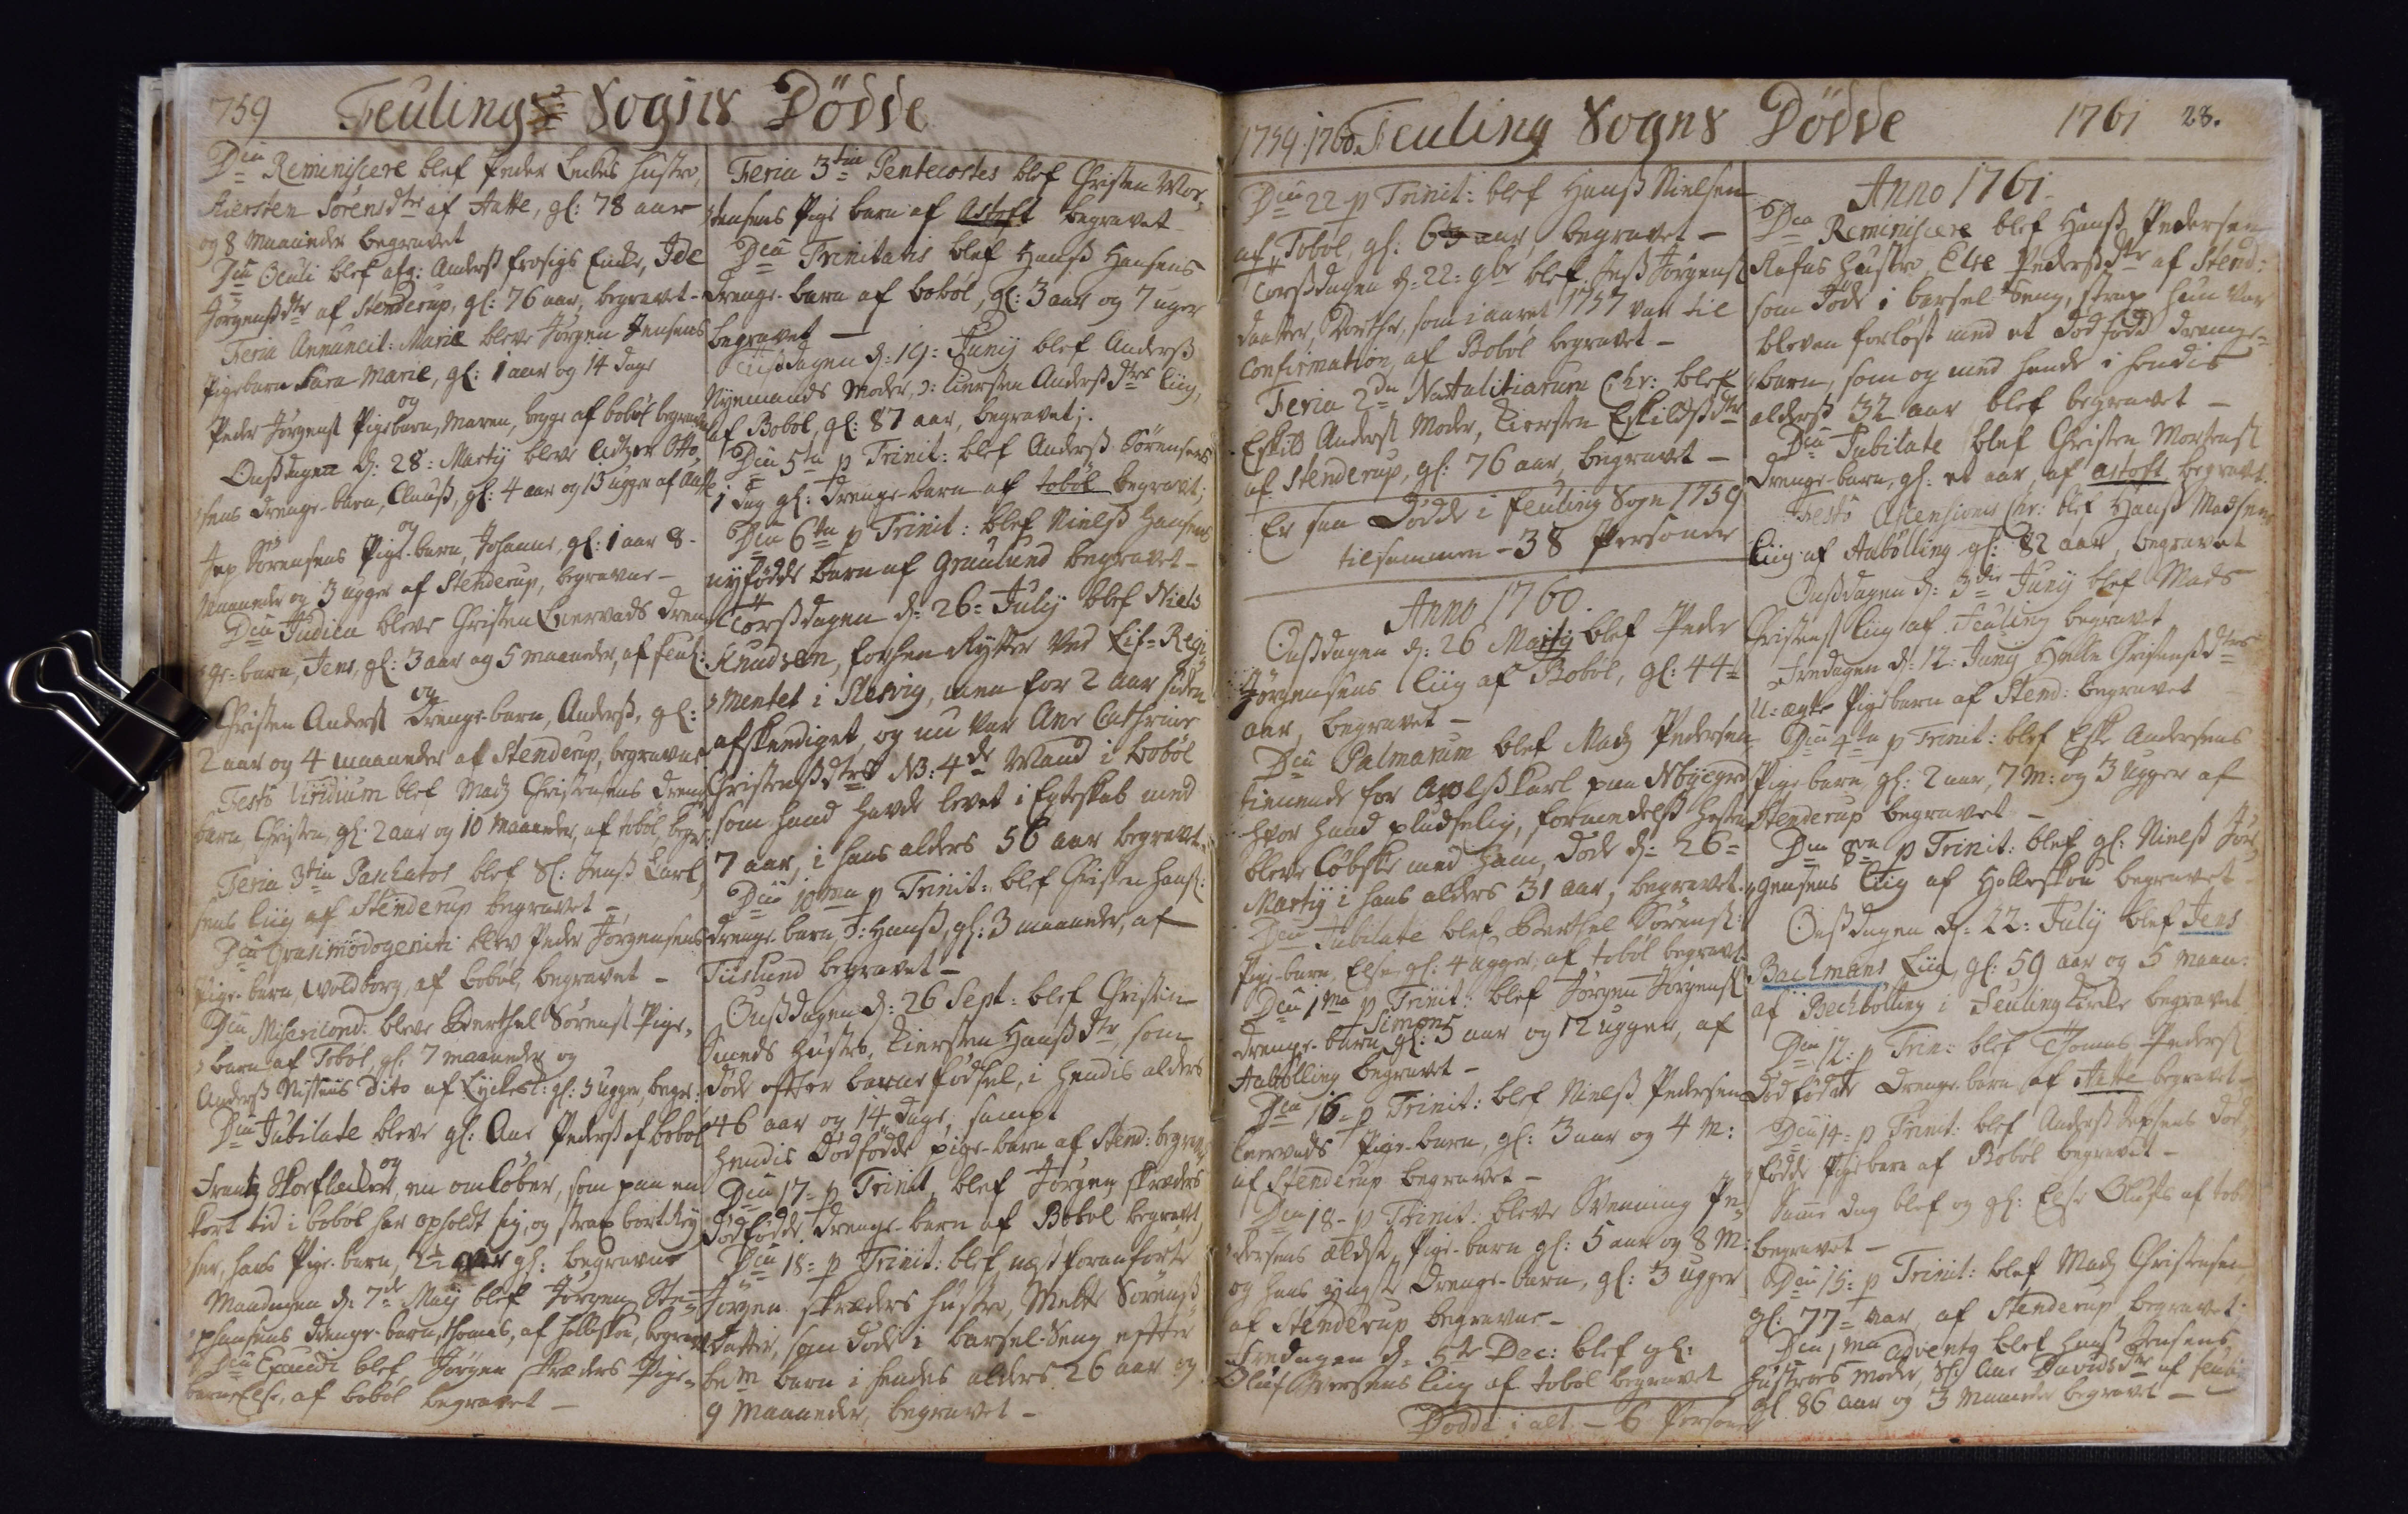1759 
Feulings  Sogns Dødde
Dca Reminiscere blef Peder Leckes Hustro
Kiersten Sørensdtr af Aatte, gl: 78 aar
og 8 Maaneder begravet
Dca Oculi blef afg: Anderss Frosigs Encke, Ide
Jørgensdtr af Stenderup, gl: 76 aar. begravet -
Feria Annuncit: Mariæ bleve Jørgen Jensens
Pigebarn Sara Marie, gl: 1 aar og 14 dage
og
Peder Jørgens Pigebarn, Maren, begge af bobøl begravne
Onssdagen d: 28: Martij bleve Adtzer Otto¬
sens Drenge-barn Clauss, gl: 4 Aar og 13 ugger af Aatte
og
Jep Sørensens Pige-barn, Johanne, gl: 1 aar 8 -
Maaneder og 3 ugger af Stenderup begravne -
Dca Judica bleve Christen Leervads Dren¬
ge=barn, Jens gl: 3 aar og 5 Maaneder, af Feul:
og
Christen Anders Drenge-barn Anderss, gl:
2 Aar og 4 maaneder af Stenderup begravne
Festo Viridium blef Madz Christensens Drenge
barn, Christen, gl: 2 Aar og 10 Maaneder, af tobøl begr:
Feria 3tia Paschatos blef Sl: Jens Carl
sens Liig af Stenderup begravet
Dca Qvasimodogeniti blev Peder Jørgensens
Pige-barn, Voldborg, af bobøl begravet -
Dca Misericord: bleve Berthel Sørens Pige¬
= barn af Tobøl, gl: 7 maaneder og
Anders Nissens Dito af Lyckesk: gl: 5 ugger, begr:
Dca Jubilate bleve gl: Ane Peders af bobøl
og
Frantz Skoeflecker en omløber, som paa en
kort tid i bobøl har opholdt sig, og strax bort rey
ser, hans Pige-barn, 2½ Aar gl: begravne
Mandagen d: 7de Maij blef Jørgen Ste¬
phansens Drenge-barn, Thomas, af Holleskov, begrav##
Dca Exaudi blef Jørgen Skræders Pige.¬
barn Else af bobøl begrafvet
Feria 3tia Pentecostes blef Christen Mor¬
tensens Pige barn af Astoft begravet
Dca Trinitatis blef Hanss Hansens
Drenge-barn af bobøl, gl: 3 Aar og 7 Uger
begravet -
Tiissdagen d: 19: Junij blef Anderss
Nyemands Moder, ɔ: Kiersten Anderssdtr Liig,
af Bobol, gl: 87 aar begravet;.
Dca 5ta p Trinit: blef Anders Sørensens
1 Dag gl: Drenge-barn af tobøl begravet;
Dca 6ta p Trinit: blef Niels Hansens
nyfødde barn af Graulund begravet -
Torssdagen d: 26: Julij bleff Niels
Knudsen, forhen Rytter ved Lif=Regi¬
mentet i Slevig, men for 2 Aar siden
afskendiget, og nu var Ane Cathrine
Christenssdtr NB: 4de Mand i Bobøl
som hand havde levet i Egteskab med
7 aar, i hans alders 56 aar begravet.
Dca 10ma p Trinit: blef Christen Hanss:
drenge-barn ɔ: Hanss, gl: 3 maaneder, af
Tiislund begravet -
Onssdagen d: 26 Sept: bleff Christen
Smeds Hustro, Kiersten Hanssdtr som
døde efter barnefødsel, i hendes alders
46 aar og 14 dage, sampt
hendis dødfødde pige-barn af Stend: begravne
Dca 17: p Trinit blef Jørgen Skræders
dødfødde Drenge-barn af Bobol begravet
Dca 18: p Trinit: blef næst foranførte
Jørgen skræders hustru, Mette Sørenss¬
datter, som døde i Barsel-Seng efter
bem barn i hendes alders 26 aar og
9 Maaneder, begravet -
1759 1760 Feuling Sogns Dødde
Dca 22 p Trinit: bleff Hanss Nielsen
af Tobøl, gl: 63 aar begravet -
Torssdagen d: 22: 9br blef Jens Jørgens
daatter, Dorthe, som i Aaret 1757 var til
Confirmation af Bobøl begravet -
Feria 2da Natalitiatum Chr: blef
Eskild Anders Moder Kiersten Eskildssdtr
af Stenderup, gl: 76 aar begravet -
Er saa Dødde i Feuling Sogn 1759
tilsammen - 38 Personer
Anno 1760.
Onssdagen d: 26 Martij blef Peder
Jørgensens Liig af Bobøl, gl: 44 =
aar, begravet -
Dca Palmarum blef Madz Pedersen
tienende for Avelskarl paa Nbyegrd
hvor hand pludselig, formedelss hestene
bleve løbske med ham, døde d= 26=
Martij i hans alders 31 Aar, begravet.
Dca Jubilate bleff Berthel Sørenss:
Pige-barn Else gl: 4 ugger af tobøl begravet.
Dca 1ma p Trinit: blef Jørgen Jørgens
Simons
drenge-barn ^ gl: 5 Aar og 12 ugger af
Aabølling begravet -
Dca 16 - p Trinit: blef Niels Pedersens
Leervads Pige-barn, gl: 3 Aar og 4 m:
af Stenderup begravet
Dca 18 - p Trinit: bleve Svenning Pe¬
= dersens ældste Pige-barn gl: 5 Aar og 8 m
og hans yngste Drenge-barn, gl: 3 Ugger
af Stenderup begravne -
Fredagen d: 5te Dec: blef gl:
Oluf Iversens liig af tobol begravet
Dødde i alt - 6 Personer
1761 28.
Anno 1761
Dca Reminiscere blef Hanss Pedersen
Rafns Hustru, Else Pederssdtr af Stend:
som døde i barsel=Seng, strax hun var
bleven forløst med et dødfødd drenge¬
= barn, som og med hende i hendis
alders 32 Aar blef begravet -
Dca Jubilate blef Christen Mortens
drenge-barn gl: et aar, af Astoft begrafvet.
Festo Ascensionis Chr: blef Hans Madsens
Liig af Aabølling gl: 82 aar, begravet
Onssdagen d: 3de Junij blef Mads
Christens liig af Feuling begravet
Fredagen d: 12: Junij Helle Christenssdtr
U=ægte Pige barn af Stend: begravet.
Dca 4ta p Trinit: blef Eske Andersens
Pige-barn gl: 2 aar 17 M: og 3 Ugger af
Stenderup begravet.
Dca 8va p Trinit: blef gl: Nielss Jør¬
gensens Liig af Holleskou begravet
Onssdagen d: 22: Julij blef Jens
Bachmans Liig gl: 59 aar og 5 maan:
af Bechbølling i Feuling Kirke begravet
Dca 12: p Trin: blef Thomas Peders
dødfødde Drenge-barn af Aatte begravet.
Dca 14: p Trinit: blef AnderSs Jepsens død¬
fødde Pigebarn af Bobøl begravet -
Samme Dag blef og gl: Else Olufs af tobøl
begravet -
Dca 15: p Trinit: blef Madz Christensen
gl: 77 = Aar af Stenderup begravet
Dca 1ma Adventy bleff Hans Jensens
Hustroes Moder, Sl: Ane Davidsdtr af Feuling
gl 86 Aar og 3 Maaneder begravet -
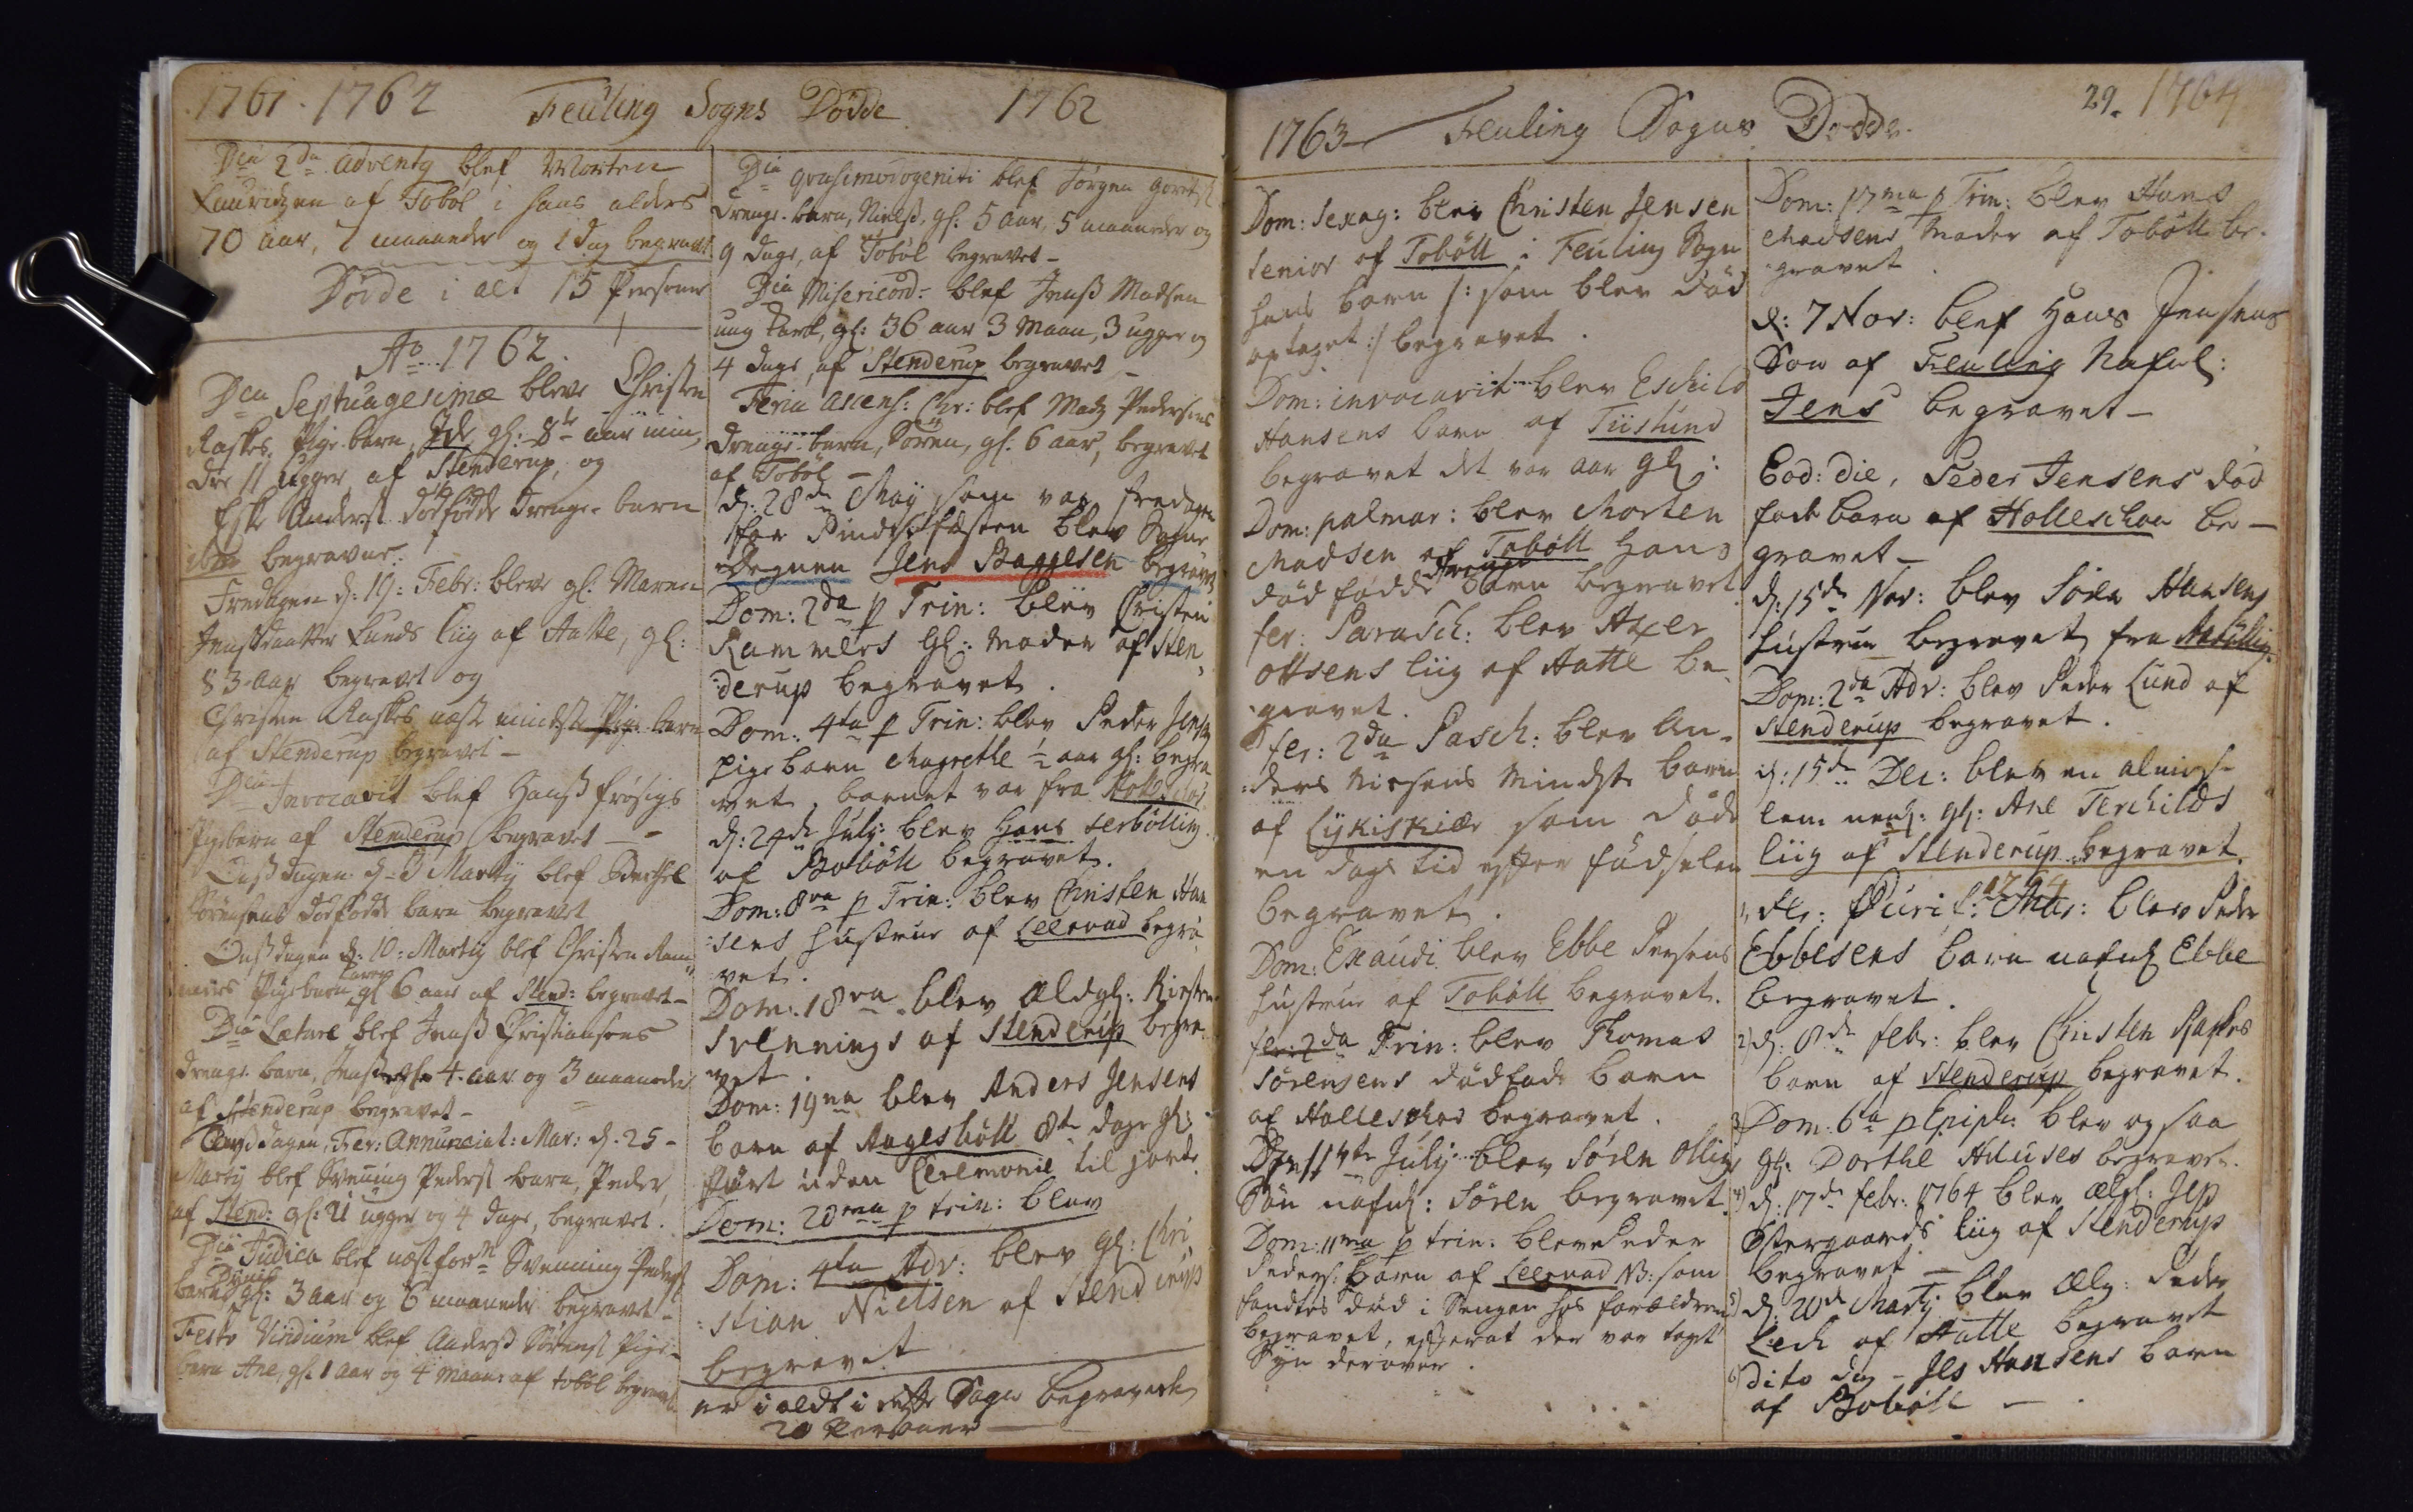1761. 1762
Felling Sogns Dødde.
Dca 2da Adventy blef Morten
Lauritzøn af Tobøl i hans alders
70 aar, 7 maaneder og 1 dag begravet
Dødde i alt 15 Personer
Ao 1762
Dca Septuagesimæ bleve Christen
Raskes Pige-barn, Ide gl: 8te aar min
dre 11 ugger af Stenderup, og
Eske Anders dødfødde Drenge-barn.
ibm begravne
Fredagen d: 19: Febr: bleve gl: Maren
Jenssdaatter Lunds Liig af Aatte, gl:
83 Aar begravet og
Christen Raskes næste mindste Pige-barn
af Stenderup begravet -
Dca Invocavit blef Hanss Frøsigs
Pigebarn af Stenderup begravet.
Onssdagen d: 3 Martij blef Berthel
Sørensens dødfødde barn begravet
Onssdagen d: 10: Martij blef Christen Ram¬
Karen
mers Pigebarn ^ gl 6 aar af Stend: begravet.
Dca Lætare blef Jenss Christiansens
drenge barn, Jenss gl: 4. aar og 3 maaneder
af Senderup begravet -
Onssdagen Fer: Annunciat: Mar: d. 25 -
Martij blef Svenning Peders barn, Peder
af Stend: gl: 21 ugger og 4 dage, begravet.
Dca Judica blef næst forn Svemming Peders
Dynis
barn ^ gl: 3 aar og 6 maaneder begravet -
Festo Viridium blef Anders Sørens Pige¬
barn Ane, gl 1 Aar og 4 Maan: af tobøl begravet
1762
Die Qvasimodogeniti bleff Jørgen Gorets
Drenge-barn Niels gl: 5 aar 5 maaneder og
9 Dage af Tobøl begravet -
Dca Misericord: blef Jens Madsen
ung Karl, gl: 36 aar 3 maan, 3 ugger og
4 Dage af Stenderup begravet
Feria Ascens. Chr: blef Matz Pedersens
Drenge-barn, Søren, gl: 6 aar, begravet
af Tobøl
d: 28de Maij som var Fredagen
for Pindsefæsten blev Sogne
Degnen Jens Baggesen begravet
Dom: 2da p Trin: blev Christen
Rammers gl: Moder af Sten¬
derup begravet
Dom: 4ta p Trin: blev Peder Jensens
pige barn Magrethe ½ aar gl: begra
vet, barnet var fra Holleschou
d: 24de Julij blev Hans serbølling
af Bobøll begravet.
Dom: 8va p Trin: blev Christen Han¬
sens Hustrue af Leervad begra
vet.
Dom: 18va blev Ældgl: Kirsten
Svennings af Stenderup begra
vet
Dom: 19na blev Anders Jensens
barn af Aagesbøll 8te dage gl:
stæet uden Ceremonie til jorde
Dom: 20ma p Trin: blev
Dom: 4ta Adv: blev gl: Chri¬
stian Nielsen af Stenderup
begravet
er i alt i dette Sogn Begravet
20 Personer -
1763.
Feuling Sogns Dødde.
Dom: Sexag: blev Christen Jensen
senior af Tobøll i Feuling Sogn
hans barn /: som blev død
optaget :/ begravet
Dom: Invocavit blev Eschild
Hansens barn af Tiislund
begravet det var aar gl:
Dom: Palmar: blev Morten
Madsen af Tobøll Hans
drenge
dødfødde barn begravet
Fer: Paasch. blev Aner
Olsens lig af Matte be¬
gravet
Fer: 2da Parasch: blev An¬
dere Nissens mindste barn
af Lykiskiær som døde
en da tid efter Fødselen.
begravet
Dom: Exaudi blev Ebbe Jensens
Hustrue af Tobøll begravet.
Fer: 2da Trin: blev Thomas
Sørensens dødfode Barn
af Holleschou begravet
Dom 11##te Julij blev Søren Ollings
Søn nafnl: Søren begravet
Dom: 11ma p Trin: blev Peder
Peders Barn af Leervad NB: som
fandtes død i Sengen hos forældrene
begravet, efter at der var taget
Syn derover
29.
1764
Dom: 17ma p Trin: blev Hans
Madsens Moder af Tobøl be¬
gravet
d: 7 Nov: blef Hans Jensens
Søn af Feuling Nafnl:
Jens begravet -
Eod: Die, Peder Jensens død
fode Barn af Holleschou be¬
gravet -
d: 15de Nov: blev Søren Hansens
Hustrue begravet fra Aabølling
Dom: 2da Adv: blev Peder Lund af
Stenderup begravet
d: 15de Dec: blev en almisse
lem ne##: gl: Ane Terchilds
Liig af Stenderup begravet
1) Fer: Purif: Mar: blev Peder
Ebbesens barn nafnl Ebbe
begravet
2) d: 8de Febr: blev Christen Raskes
Barn af Stenderup begravet
3) Dom: 6ta Epiph: blev ogsaa
gl: Dorthe Auuses begraven
4) d: 17de Febr: 1764 blev ælgl: Jep
Østergaards Liig af Stenderup
begravet
5) d: 20de Martij blev ælg: Peder
Lech af Aatte begravet
6) dito dag - og Jes Hansens barn
af Bobøll